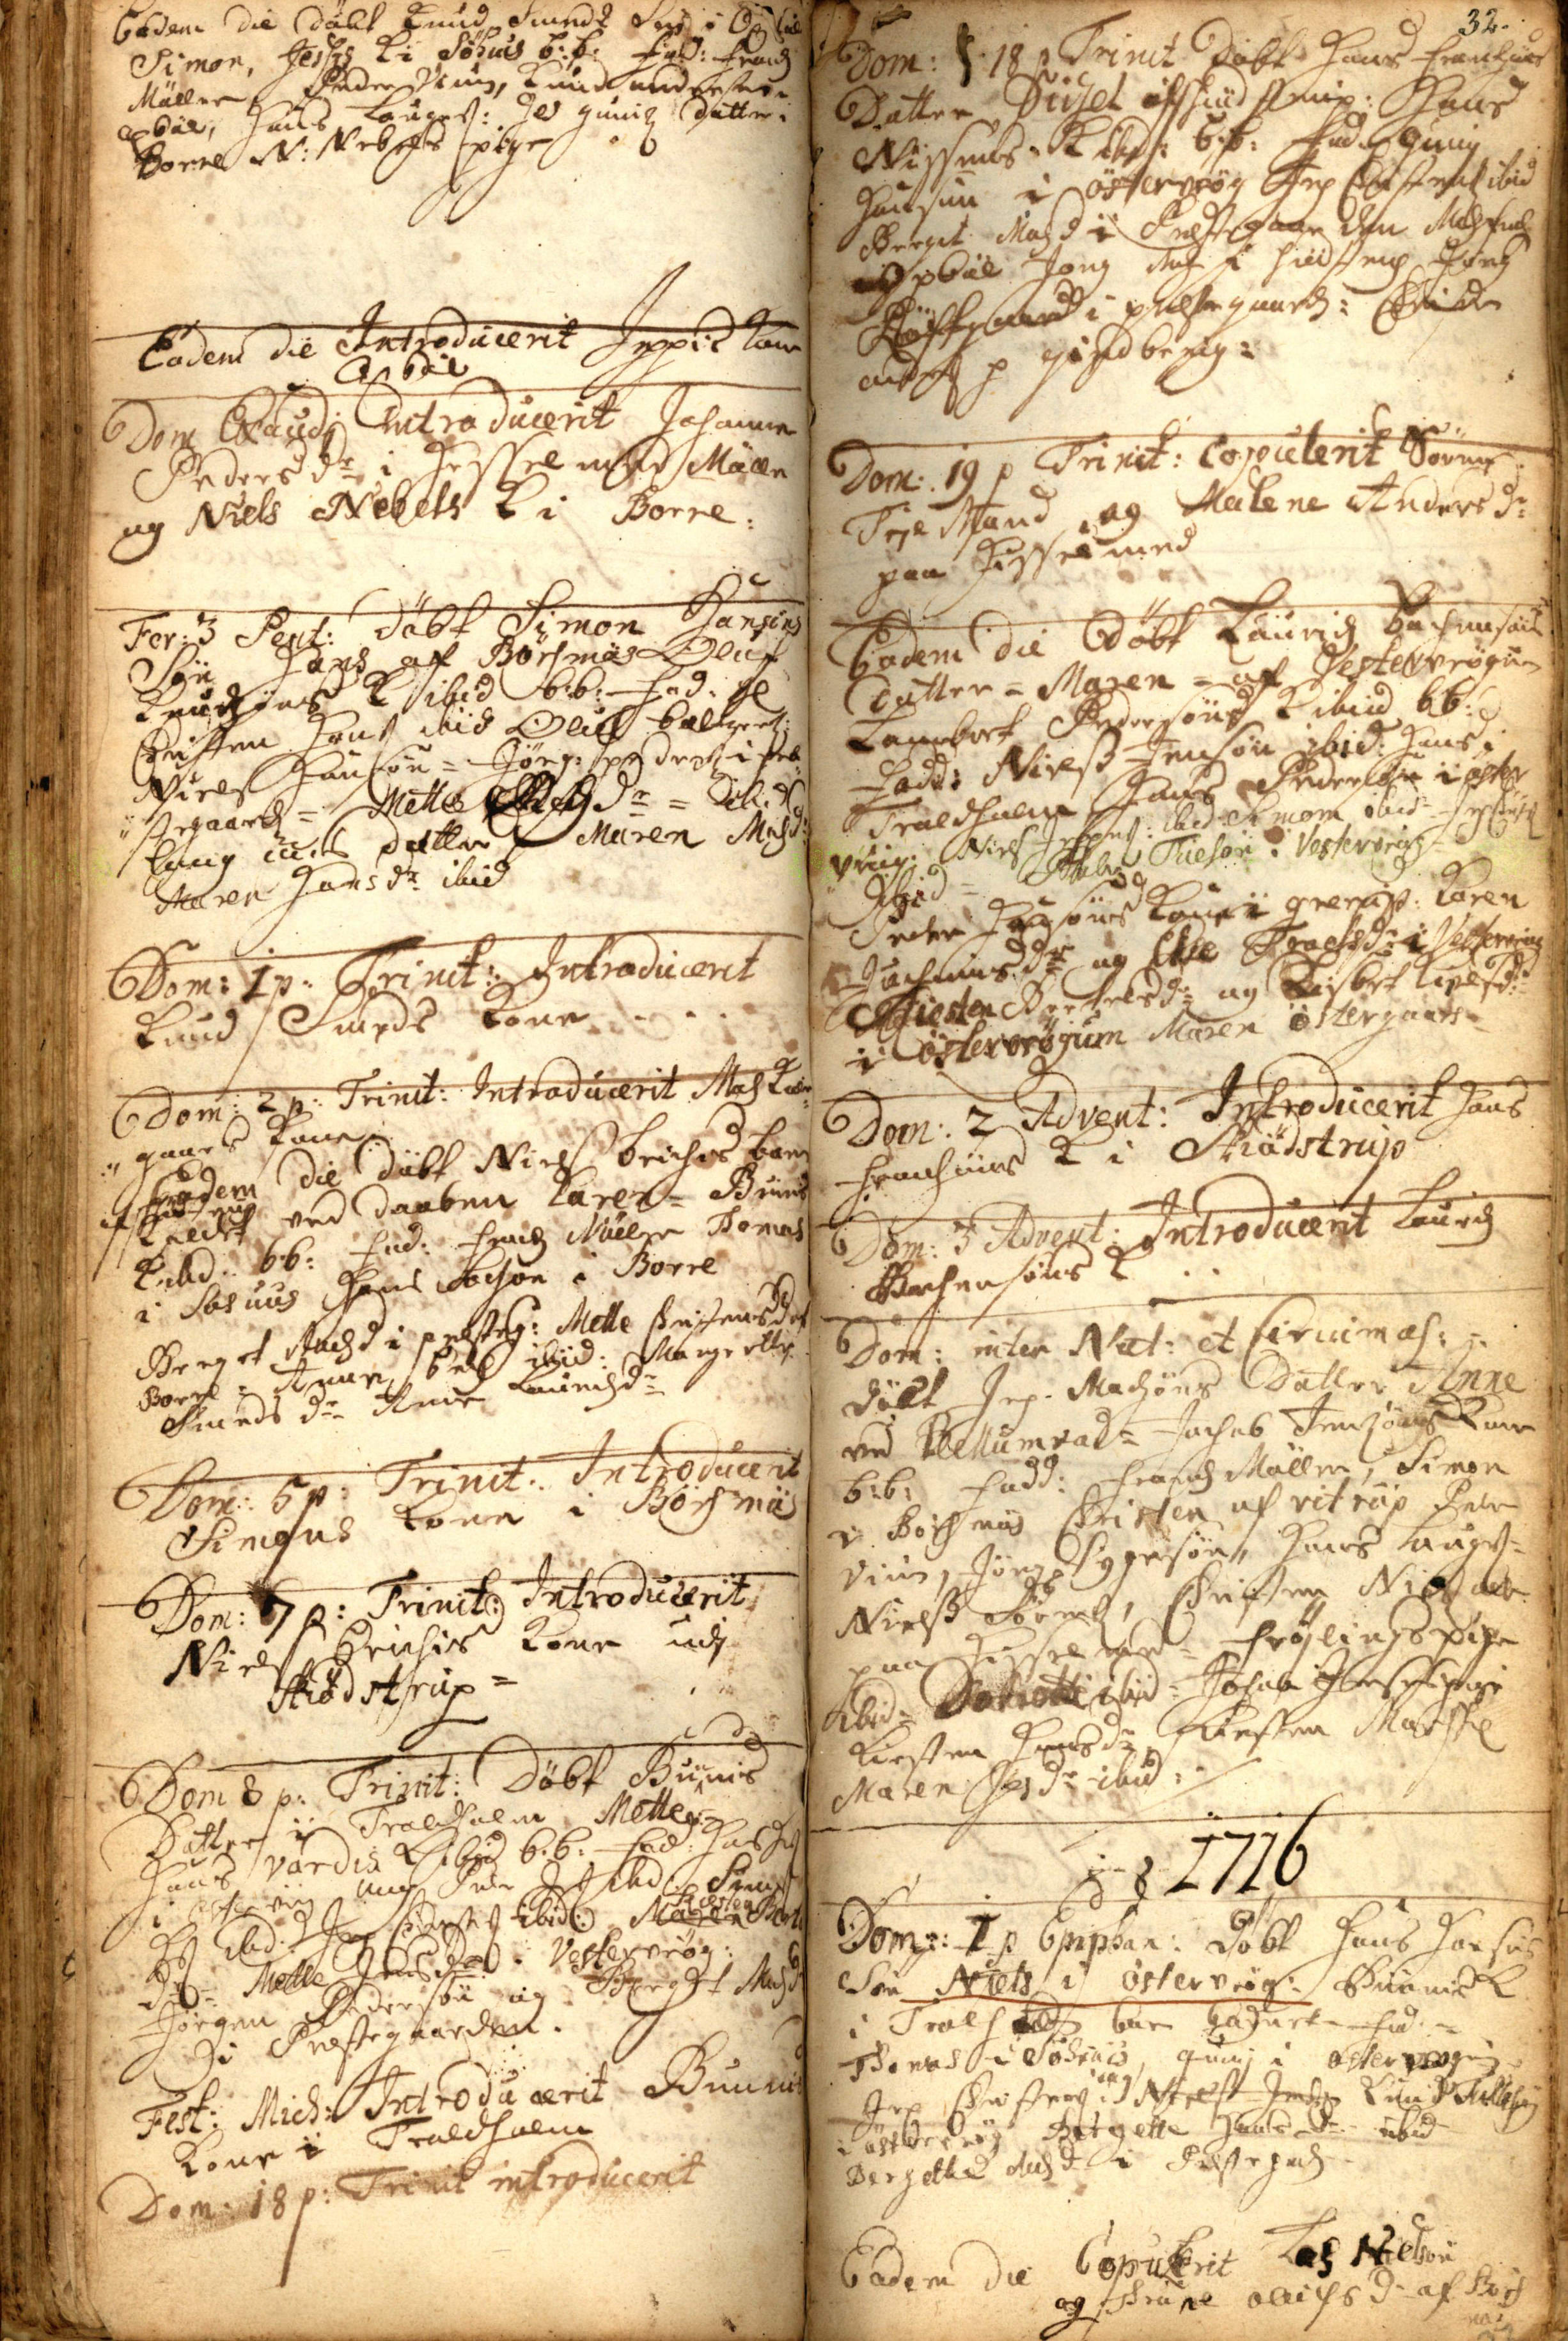Eodem Die Døbt Knud Smedz Søn i Oxbøl
Simon, Jessis K i Søhuus b:b: Fad: Frandz
Møller, Peder Vium, Knud Andersen i
Oxbøl, Hans Lauges: Jes Gunniz Datter i
Borre N.Nebels pige
Eodem Die Introducerit Jeppis Kone
i Oxbøl
Dom. Exaudi Introducerit Johanne
Pedersdr i Hisselmed Mølle
og Niels Nebels K i Borre:
Fer: 3 Pent: Døbt Simon Hansøns
Søn Hans af Børsmøs Oluf
Knudzøns K ibid b:b: Fad: gl
Christen Hans ibid Oluf Baltzers:
Niels Hansøn= Jørgen Peders i Præ¬
stegaard= Mette ##dr= ##
## ## Datter, Maren Madzd:
Maren Hansdr ibid
Dom: 1 p Trinit: Introducerit
Knud Smeds Kone
Dom: 2 p: Trinit: Introducerit Madz Kiær¬
gaards Kone
Eodem Die Døbt Niels Briches Barn
af Schiødstrup
kaldet ved Daaben Karen= Bunnis
Enke## b:b: Fad: Frandz Møller Thomas
i Søhuus Hans Lassøn i Borre
Berget And##d i Præsteg: Mette Christensd af
Borre= Anne B## ibid: Margrette
Suends## dr,Anne Lauridzdr##
Dom: 5 p: Trinit: Introducerit
Simons Kone i Børsmøs
Dom: 7 p: Trinit: Introducerit
Niels Brichis Kone udj
Skiødstrup=
Dom 8 p: Trinit: Døbt Bunnis
Datter i Troldholm Mette
Hans Vardis K ibid b:b: Fad: Hans H##
i Østervrøg ung Peder Jes ibd, S##
Kiesten
H## ibd: Jep Christens ibid:, Maren Be##
dr Mette Jensdr i Vestervrøg:
Jørgen Pedersøn og Bergit Madzd
i Præstegaarden.
Fest. Mich. Introducerit Bunnis
Kone i Troldholm
Dom: 18 p: Trinit introducerit
32
Dom.18 p Trinit Døbt Hans Frandzøns
Datter Sidzel af Schiødstrup: Hans
Nissens K ibid: b:b.: Fad: Guni##
Hansøn i Østervrøg., Jep Christensen ibid
Bergit Madzd i Præstegaarden, Madz F##
Oxbøl, Jorg M## i Schiødstrup Jørg
Løftegaard i Præstegaard= Christen
Anders p Giedberig=
Dom: 19 p Trinit: Copuelerit Søren
Tejl Mand og Malene Andersdr
paa Hisselmed
Eodem Die Døbt Lauridz Bachensøns
Datter= Maren af Vestervrøgum
Lambert Pedersøns K ibid bb:
Fadd: Niels Jensøn ibid: Hans i
Troldholm Hans Pedersøn i Øster
vrøg: Niels Jesppes: ibid: Simon ibid J##
ibid= Peder## Thuesøn i Vestervrøg=
Peder Hansøns Kone i Grerup: Karen
Juchomisdr og Else Troelsdr i Vestervrøg
Kiesten Bertelsdr og Lisbet Kielsdr
i Østervrøgum Maren i Østergaars.
Dom: 2 Advent: Introducerit Hans
Frandzøns K i Skiødstrup
Dom: 3 Advent Introducerit Lauridz
Bachensøns K
Dom: inter Nat: et Circumces:
Døbt Jep Madzøns Datter Anne
ved Bellumvad= Jachob Jensøns Kone
b:b: Fadd: Frandz Møller, Simon
i Børsmøs Christen af Vitrup Peder
Vium Jørg Tygesøn, Hans Lauges=
Niels Sørens, Christen Ni## ##
paa Hisselmed= frøjylings## pige
ibid= Dorrette ibid= Johan Jensens pige
Kirsten Handr Kirsten Marsch
Maren Jesdr ibid.
## 1716
Dom: 1 p Epiphan: Døbt Hans Hansøns
Søn Niels i Østervrøg: Bunnis K.
i Troldholm## bar Barnet Fad:
Thomas i Søhuus Gunij i Østervrøgum##
Jep Christens Niels Jens Knud ##
i Østervrøg Bergette Hansdr ibid
Bergette Madzd## i Præstegrd
Eodem Die Copulerit Las Nielsøn
og Thrine## Olufsd af Børs
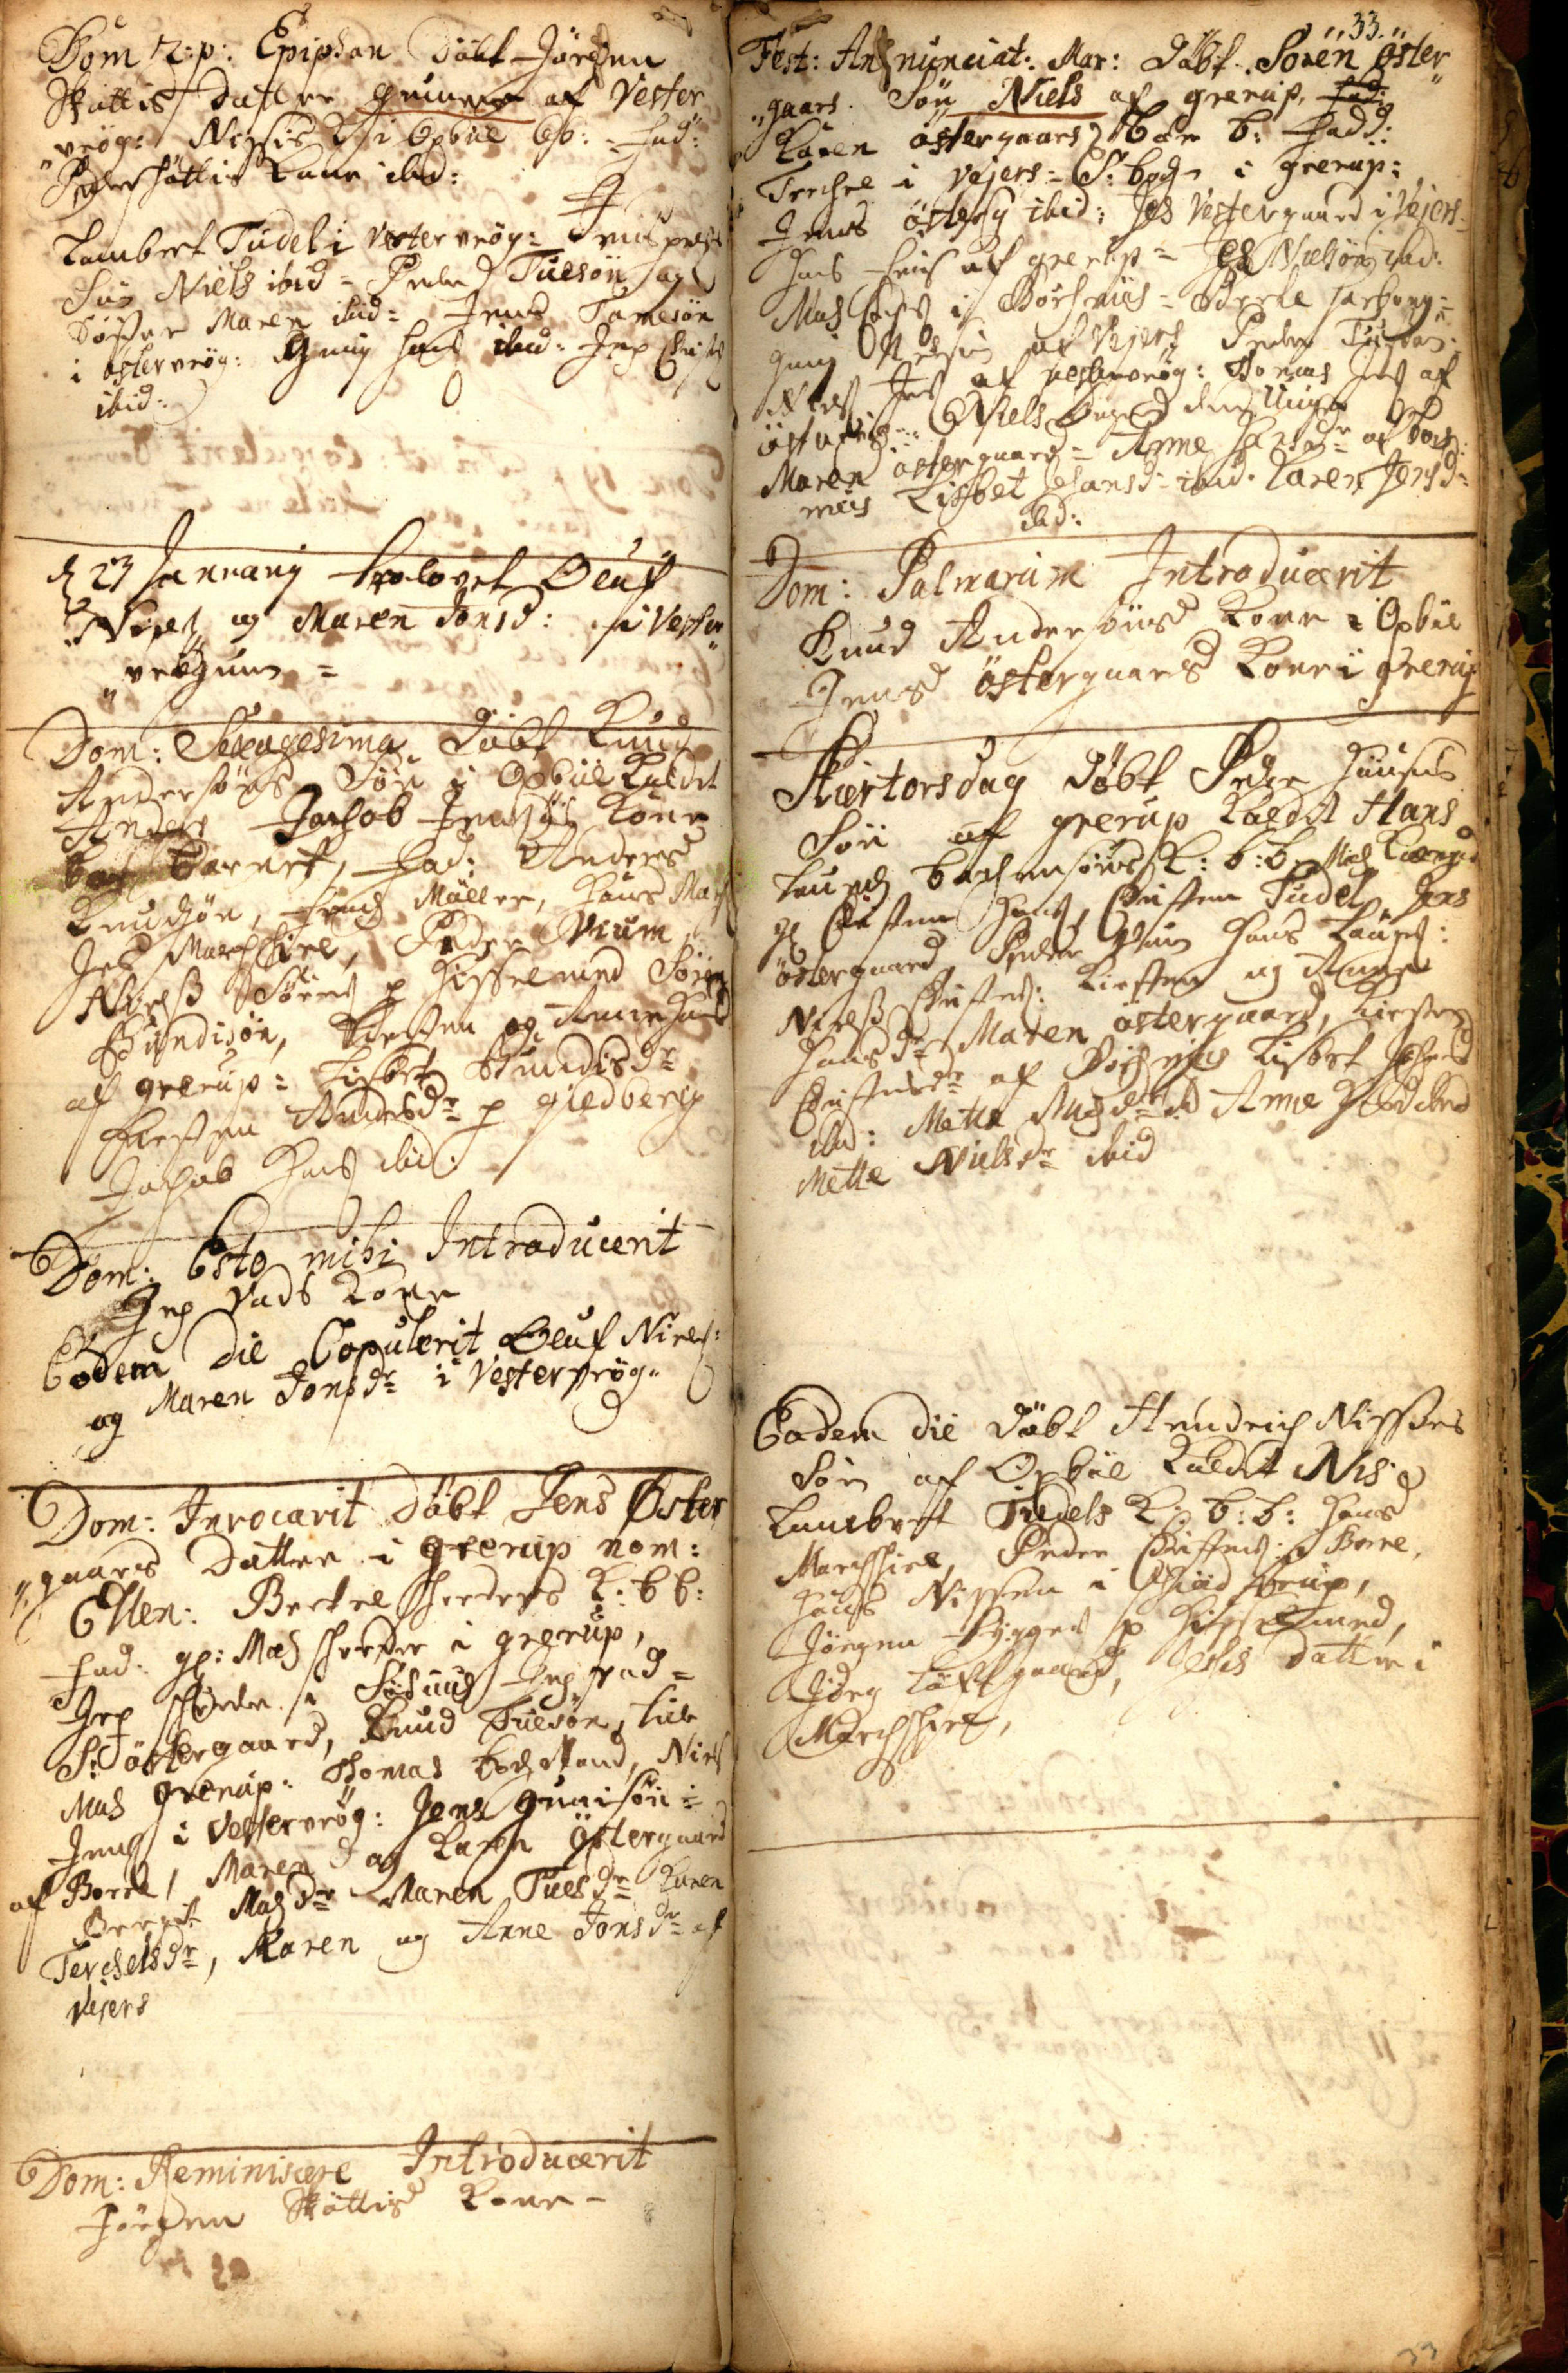Dom 2: p: Epiphan. Døbt Jørgen
Skøttis Datter Gunner af Vester
vrøg: Nissis K i Oxbøl bb:= Fad:
Peder Schøttis Kone ibid:
Lambert Tudel i Vestervrøg= Iver## Pedersens##
Søn Niels ibid= Peder Tuesøn og
Søster Maren ibid= Jens Tomesøn
i Østervrøg: Gunij Hans ibid: Jep Christens
ibid:
d 23 Januarij trolovet Oluf
Niels og Maren Jonsd: i Vester¬
vrøgum=
Dom. Sexagesima Døbt Knud
Andersøns Søn i Oxbøl kaldet
Anders Jachob Jensøns Kone
bar Barnet, Fad: Anders
Knudzøn, Frandz Møller, Hans Mars
Jes Marchschiel, Peder Vium
Niels Sørens p Hisselmed Søren
Bundisøn, Kirsten og Anne Hansd
af Grerup= Lisbet Bundisdr
Kiesten Andersdr p Giedberig
Jachob Hansen ibid:
Dom: Esto mihi Introducerit
Jep Vads Kone
Eodem Die Copulerit Oluf Niels:
og Maren Jonsdr i Vestervrøg"
Dom: Invocavit Døbt Jens Øster
gaards Datter i Grerup nom:
Ellen: Bertel Schreders K: bb:
Fad: gl: Madz Schreder i Grerup,
Jep Schreder i Søhuus Jep Vad=
S: Østergaard, Knud Tuesøn, Lil##
Madz Grerup: Thomas Bodz Mand, Niels
Jens i Vestervrøg: Jens Gunisøn:
af Borre, Maren og Karen Østergaard
Beret Madzdr Maren Tuesdr Karen
Terchelsdr, Karen og Anne Jonsdr af
Vejers
Dom. Reminiscere Introducerit
Jørgen Skøttis Kone-
33
33
Fest: Annunciat: Mar. Døbt Søren Øster¬
,, gaars Søn Niels af Grerup, fad.
Karen Østergaars bar b: Fadd:
Terchel i Vejers= S: Bods i Grerup:
Jens Østerg ibid: Jes Vestergaard i Vejers.
Hans Friis af Grerup= Jes Nieslsøn ibid:
Madz ## i Børsmøs= Bertel Harborg=
Gunij N## af Vejers Peder Tuesøn
Niels Jens af Vestervrøg: Thomas Jens af
Østervrøg: Niels Degn den Unge
Maren Østergaard= Anne Hansdr af Bors¬
møs Lisbet Johansd= ibid. Karen Jensdr
ibid:
Dom. Palmarum Introducerit
Knud Andersøns Kone i Oxbøl
Jens Østergaards Kone i Grerup
Skærtorsdag Døbt Peder Hansens
Søn af Grerup kaldit Hans
Lauridz Bachensøns K: b:b: Madz Kiærgd##
gl Christen Hans, Christen Tudel, Jens
Østergaard, Peder Vium, Hans Lauges:
Niels Christens: Kirsten og Anne
Hansdr Maren Østergaard, Kirsten
Christensdr af Børsmøs Lisbet Johansd##
ibid: Mette Madzdr ## Anne Hansd ibid
Mette Nielsdr ibid
Eodem Die døbt Hendrich Nissens
Søn af Oxbøl kaldet Nis
Lambert Tudels K: b:b: Hans
Marchschiel, Peder Christens i Borre,
Hans Nissen i Schiødstrup,
Jørgen Tygges p Hisselmed,
Jørg Løftgaard, Jesis Datter i
Marchschiel,